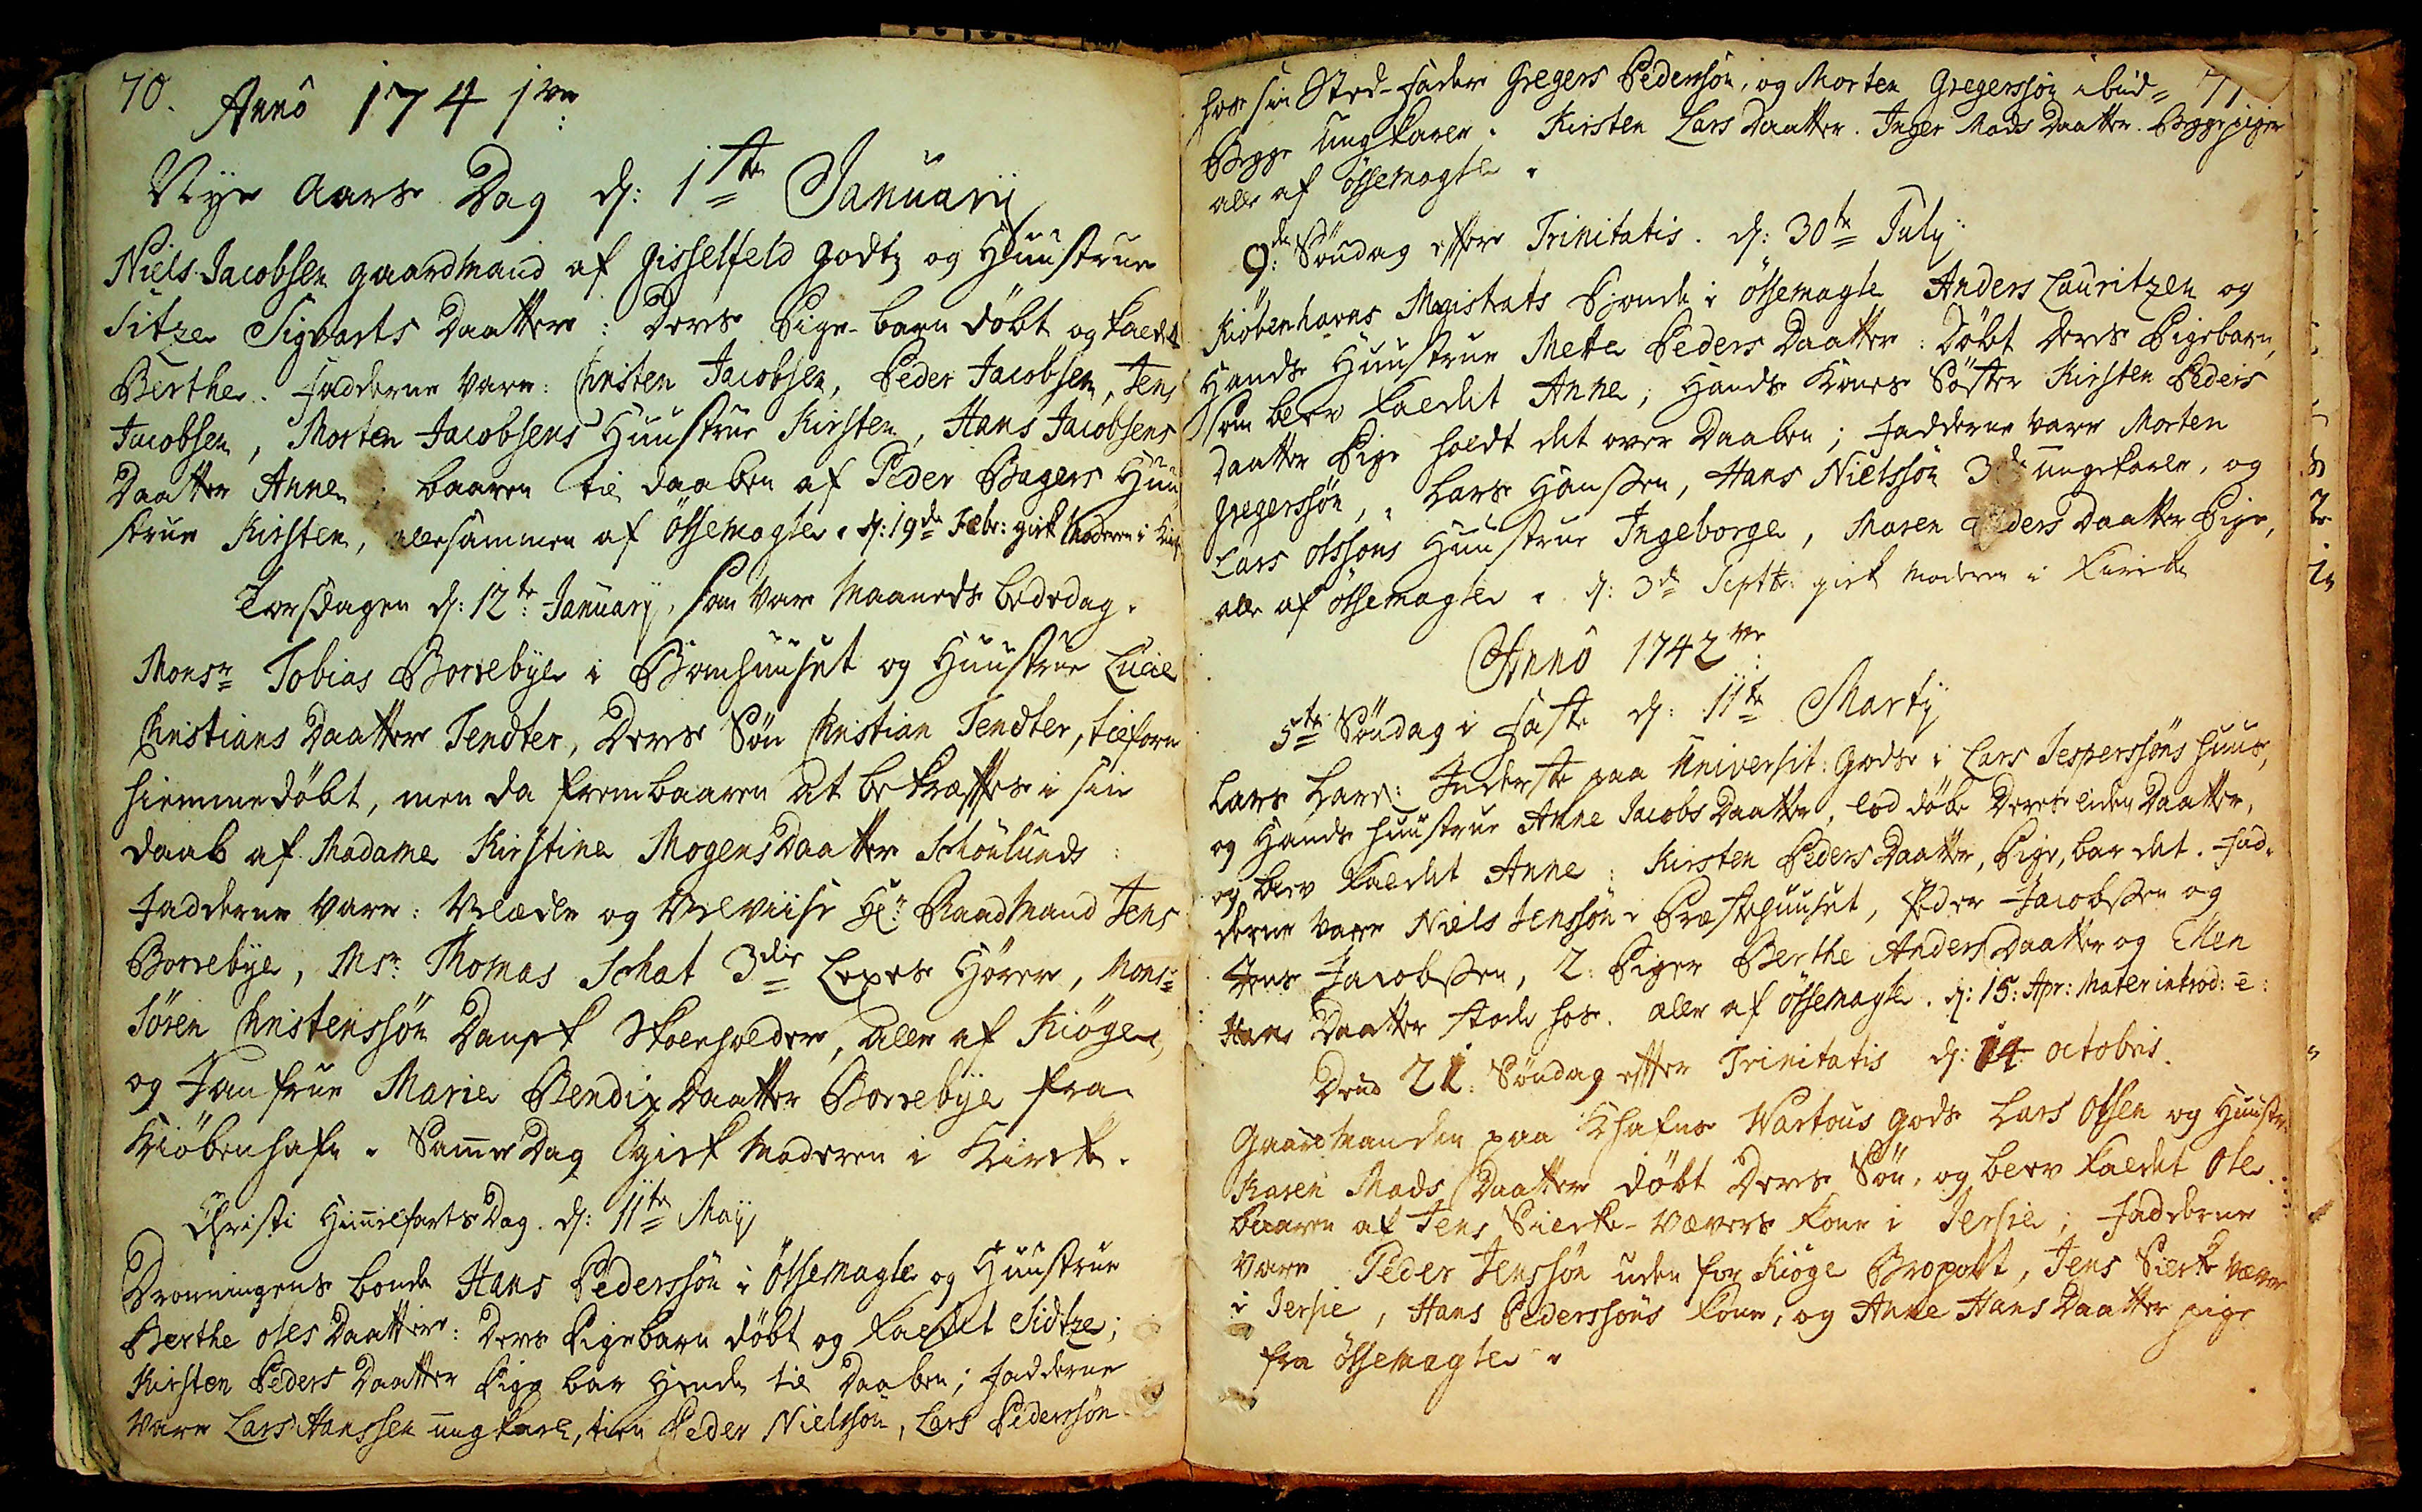70.
Anno 1741ve:
Nye Aars Dag d: 1ste Januarij
Niels Jacobsen Gaardmand af Gisselfeld Godtz og Hustrue
Sitze Sigvarts Daatter: Deres Pige-barn døbt og kaldet
Berthe. Fadderne vare: Christen Jacobsen, Peder Jacobsen, Jens
Jacobsen, Morten Jacobsens Huustrue Kirsten, Hans Jacobsens
Daatter Anne; baaren til Daaben af Peder Bagers Huu¬
strue Kirsten, allesammen af Ølsemagle. d: 19de Febr: gick Moderen i Kirke
Torsdagen d: 12te: Januarij, som var Maaneds Bededag.
Monsr Tobias Borrebye i Bomhuuset og Huustrue Lucie
Christians Daatter Tendter, Deres Søn Christian Tendter, tilforn
hiemmedøbt, men da frembaaren at bekræftes i sin
Daab af Madame Kirstine Mogens Daatter Schoulunds:
Fadderne vare: Velædle og Velviise Hr: Raadmand Jens
Borrebye, Msr: Thomas Schat 3die Lexes Hører, Monsr
Søren Christenssøn Dampf Skoleholder, alle af Kiøge,
og Jomfrue Marie Bendix Daatter Borrebye fra
Kiøbenhafn. Samme Dag gick Moderen i Kircke.
Christi Himmelfarts Dag d: 11te Maij
Dronningens Bonde Hans Pederssøn i Ølsemagle og Huustrue
Berthe Oles Daatter: Deres Pigebarn døbt og kaldet Sidtze;
Kirsten Peders Daatter Pige bar Hende til Daaben; Fadderne
vare Lars Hanssen ungkarl, tien Peder Nielssøn, Lars Pederssøn
71.
Hos sin Sted-Fader Gregers Pederssøn, og Morten Gregerssøn ibid =
Begge Ungkarle. Kirsten Lars Daatter. Inger Mads Daatter. Begge piger
Alle af Ølsemagle.
9:de Søndag efter Trinitatis. D: 30te Julij
Kiøbenhavns Magistrats Bonde i Ølsemagle Anders Lauritzen og
Hands Huustrue Mette Peders Daatter: Døbt Deres Pigebarn,
Som blev kaldet Anne; Hands Kones Søster Kirsten Peders
Daatter Pige holdt det over Daaben; Fadderne vare Morten
Gregerssøn, Lars Hanssen, Hans Nielssøn 3de Ungekarle, og
Lars Olssøns Huustrue Ingeborge, Maren Anders Daatter Pige,
alle af Ølsemagle. d: 3die Septbr: gick Moderen i Kircke
Anno 1742ve:
5te Søndag i Faste d: 11te Martij
Lars Lars: Inderste paa Universit: Gods i Lars Jesperssøns huus,
og Hands Hustrue Anne Jacobs Daatter, lod døbe Deres liden Daatter,
og blev kaldet Anne: Kirsten Peders Daatter, Pige, bar det. Fad¬
derne vare Niels Jenssøn i Præstehuuset, Peder Jacobssen og
Jens Jacobssen, 2: Piger Berthe Anders Daatter og Ellen
Hans Daatter stode hos. Alle af Ølsemagle. d: 15: Apr: Mater introd: e:
Dend 21: Søndag efter Trinitatis d: 14. octobris.
Gaardmanden paa Khafns Wartous Gods Lars Olsen og Huustr:
Karen Mads Daatter døbt Deres Søn, og blev kaldet Ole.
Baaren af Jens Sierke-Vævers Kone i Jersie; fadderne
vare Peder Jenssøn uden for Kiøge Broport, Jens Sierke Væver
i Jersie, Hans Pederssøns Kone, og Anne Hans Daatter pige
fra Ølsemagle.
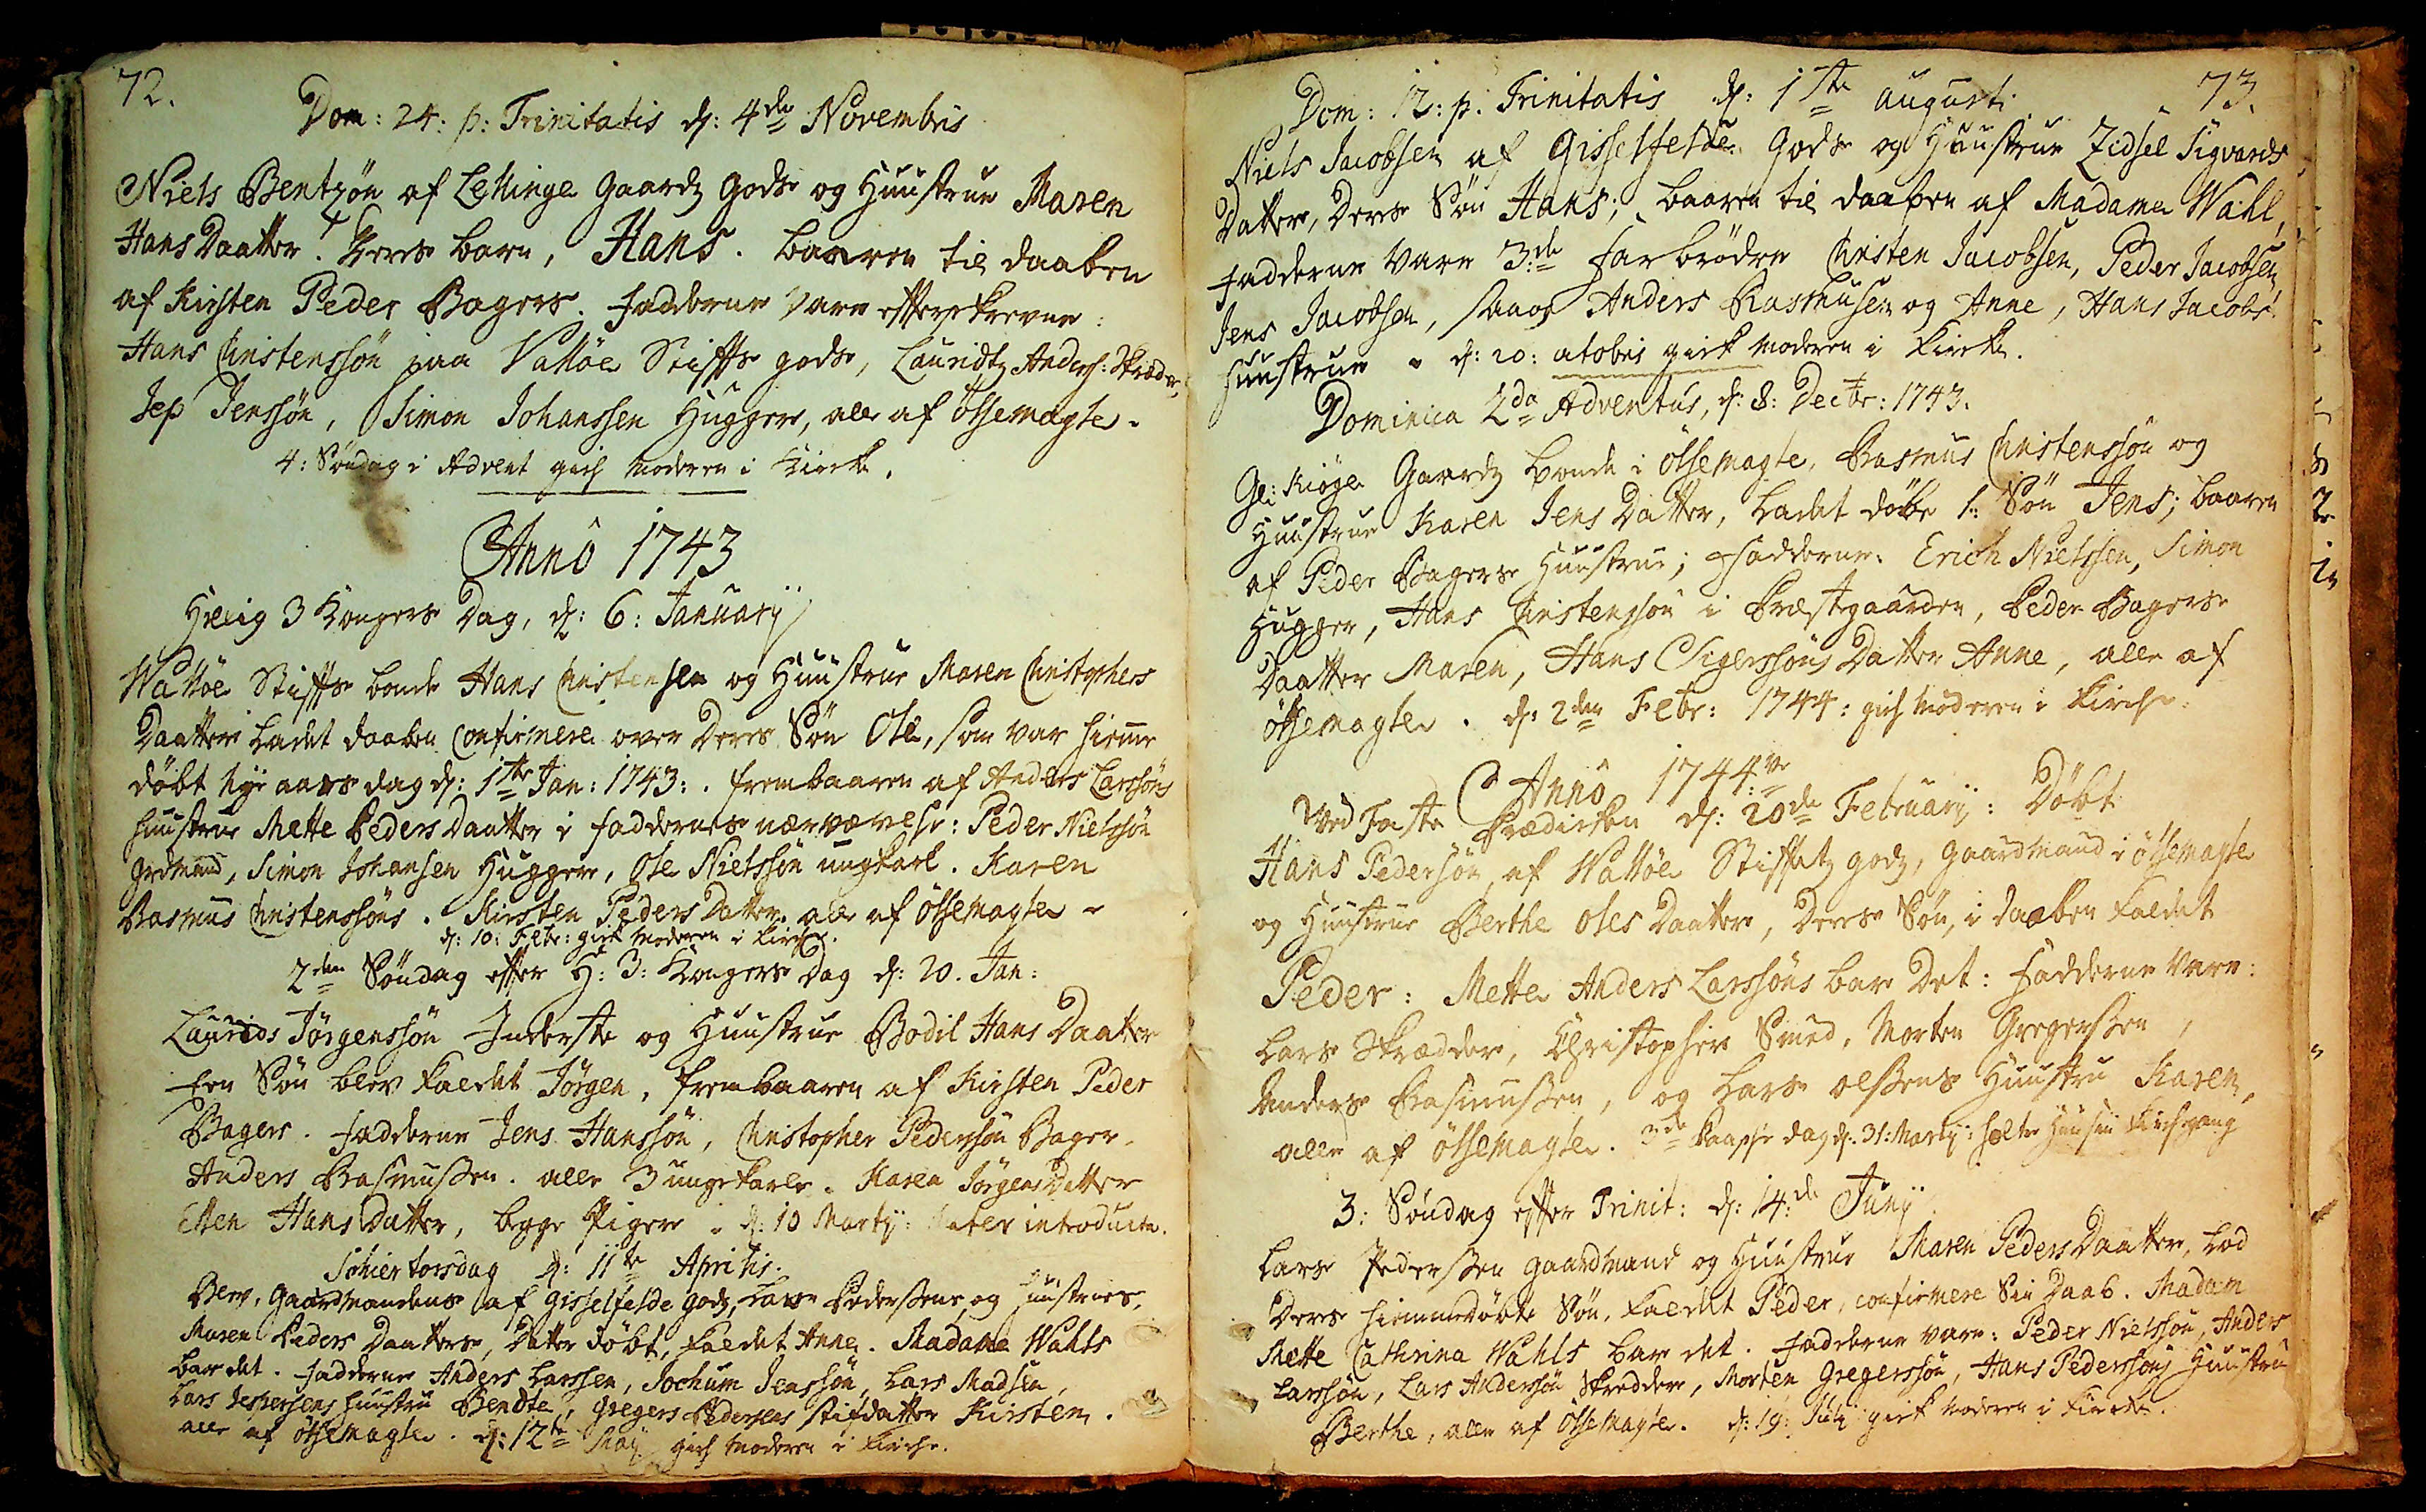72.
Dom: 24: p: Trinitatis d: 4de Novembris
Niels Bentzøn af Lellinge Gaardz Gods og Hustrue Maren
Hans Daatter. Deris Barn, Hans. Baaren til daaben
af Kirsten Peder Bagers. Fadderne vare efterskrevne:
Hans Christenssøn paa Valløe Stifts Gods, Lauridtz Anders: Skræder,
Jep Jenssøn, Simon Johanssen Hugger, alle af Ølsemagle.
4: Søndag i Advent gich Moderen i Kircke.
Anno 1743
 Hellig 3 Kongers Dag, d: 6: Januarij
Walløe Stifts Bonde Hans Christensen og Huustrue Maren Christophers
Daatter ladet Daabe confirmere over Deres Søn Ole, som var hiemme
døbt Nye aars Dag d: 1ste Jan: 1743: frembaaren af Anders Larssøns
huustrue Mette Peders Daatter i Faddernes nærværelse: Peder Nielssøn
Grdmand, Simon Johansen Hugger, Ole Nielssøn ungkarl. Karen
Rasmus Christenssøns. Kirsten Peders Datter. Alle af Ølsemagle -
d: 10: Febr: gick Moderen i Kirche.
2den Søndag efter H: 3: Kongers Dag d: 20. Jan:
Laurids Jørgenssøn Inderste og Hustrue Bodil Hans Daatter
Een Søn blev kaldet Jørgen, frembaaren af Kirsten Peder
Bagers. Fadderne Jens Hanssøn, Christopher Pedersen Bager,
Anders Rasmussen. Alle 3 ungekarle. Karen Jørgens Datter
Ellen Hans Datter, begge Piger. d: 10 Martij: Mater introducta.
Schier torsdag d: 11te Aprilis
Blev, Gaardmandens af Gisselfelde Godz, Lars Pederssens og Huustrues,
Maren Peders Daatters, Datter døbt, kaldet Anne. Madame Wahls
Bar det. Fadderne Anders Larssen, Jochum Jenssøn, Lar Madsen,
Lars Jespersens Huustrue Bendte, Gregers Pedersens stifdatter Kirsten.
Alle af Ølsemagle. d: 12te Maij gick Moderen i Kirche.
73.
Dom: 12: p: Trinitatis d: 1ste Augusti
Niels Jacobsen af Gisselfelde Gods og Huustrue Zidsel Sigvards
Datter, Deres Søn Hans; Baaren til Daaben af Madame Wahl,
Fadderne vare 3de Farbrødre Christen Jacobsen, Peder Jacobsen,
Jens Jacobsen, ## Anders Rasmusen og Anne, Hans Jacobs:
Huustrue. D: 20: octobris gick moderen i Kirke.
Dominica 2da Adventus, d: 8: Decbr: 1743.
Gl: Kiøge Gaardz Bonde i Ølsemagle, Rasmus Christenssøn og
Huustrue Karen Jens Datter, Ladet døbe 1 Søn Jens; baaren
af Peder Bagers Huustrue; Fadderne: Erich Nielssen, Simon
Hugger, Hans Christenssøn i Præstgaarden, Peder Bagers
Daatter Maren, Hans Sigerssøns Datter Anne, alle af
Ølsemagle. d: 2den Febr: 1744: gich Moderen i Kirche.
Anno 1744ve
Ved Faste Prædieken d: 20de Februarij: Døbt
Hans Pedersøn, af Walløe Stiftets Godz, Gaardmand i Ølsemagle
og Huustrue Berthe Oles Daatter, Deres Søn, i Daaben kaldet
Peder: Mette Anders Larssøns bar Det: Fadderne vare:
Lars Skrædder, Christopher Smed, Morten Gregersøn,
Anders Rasmussen, og Lars Olsens Huustru Karen,
alle af Ølsemagle. 3die Paaske dag d: 31: Marty: holte Hun sin Kirchegang
3: Søndag efter Trinit: d: 14:de Junij
Lars Pederssen Gaardmand og Huustrue Maren Peders Daatter, lod
Deres hiemmedøbte Søn, kaldet Peder, confirmere Sin Daab. Madam
Mette Cathrina Wahls bar det. Fadderne vare: Peder Nielssøn, Anders
Larssøn, Lars Anderssøn Skredder, Morten Gregerssøn, Hans Pederssøns Huustru
Berthe: alle af Ølsemagle. d: 19: July gick Moderen i Kierke.